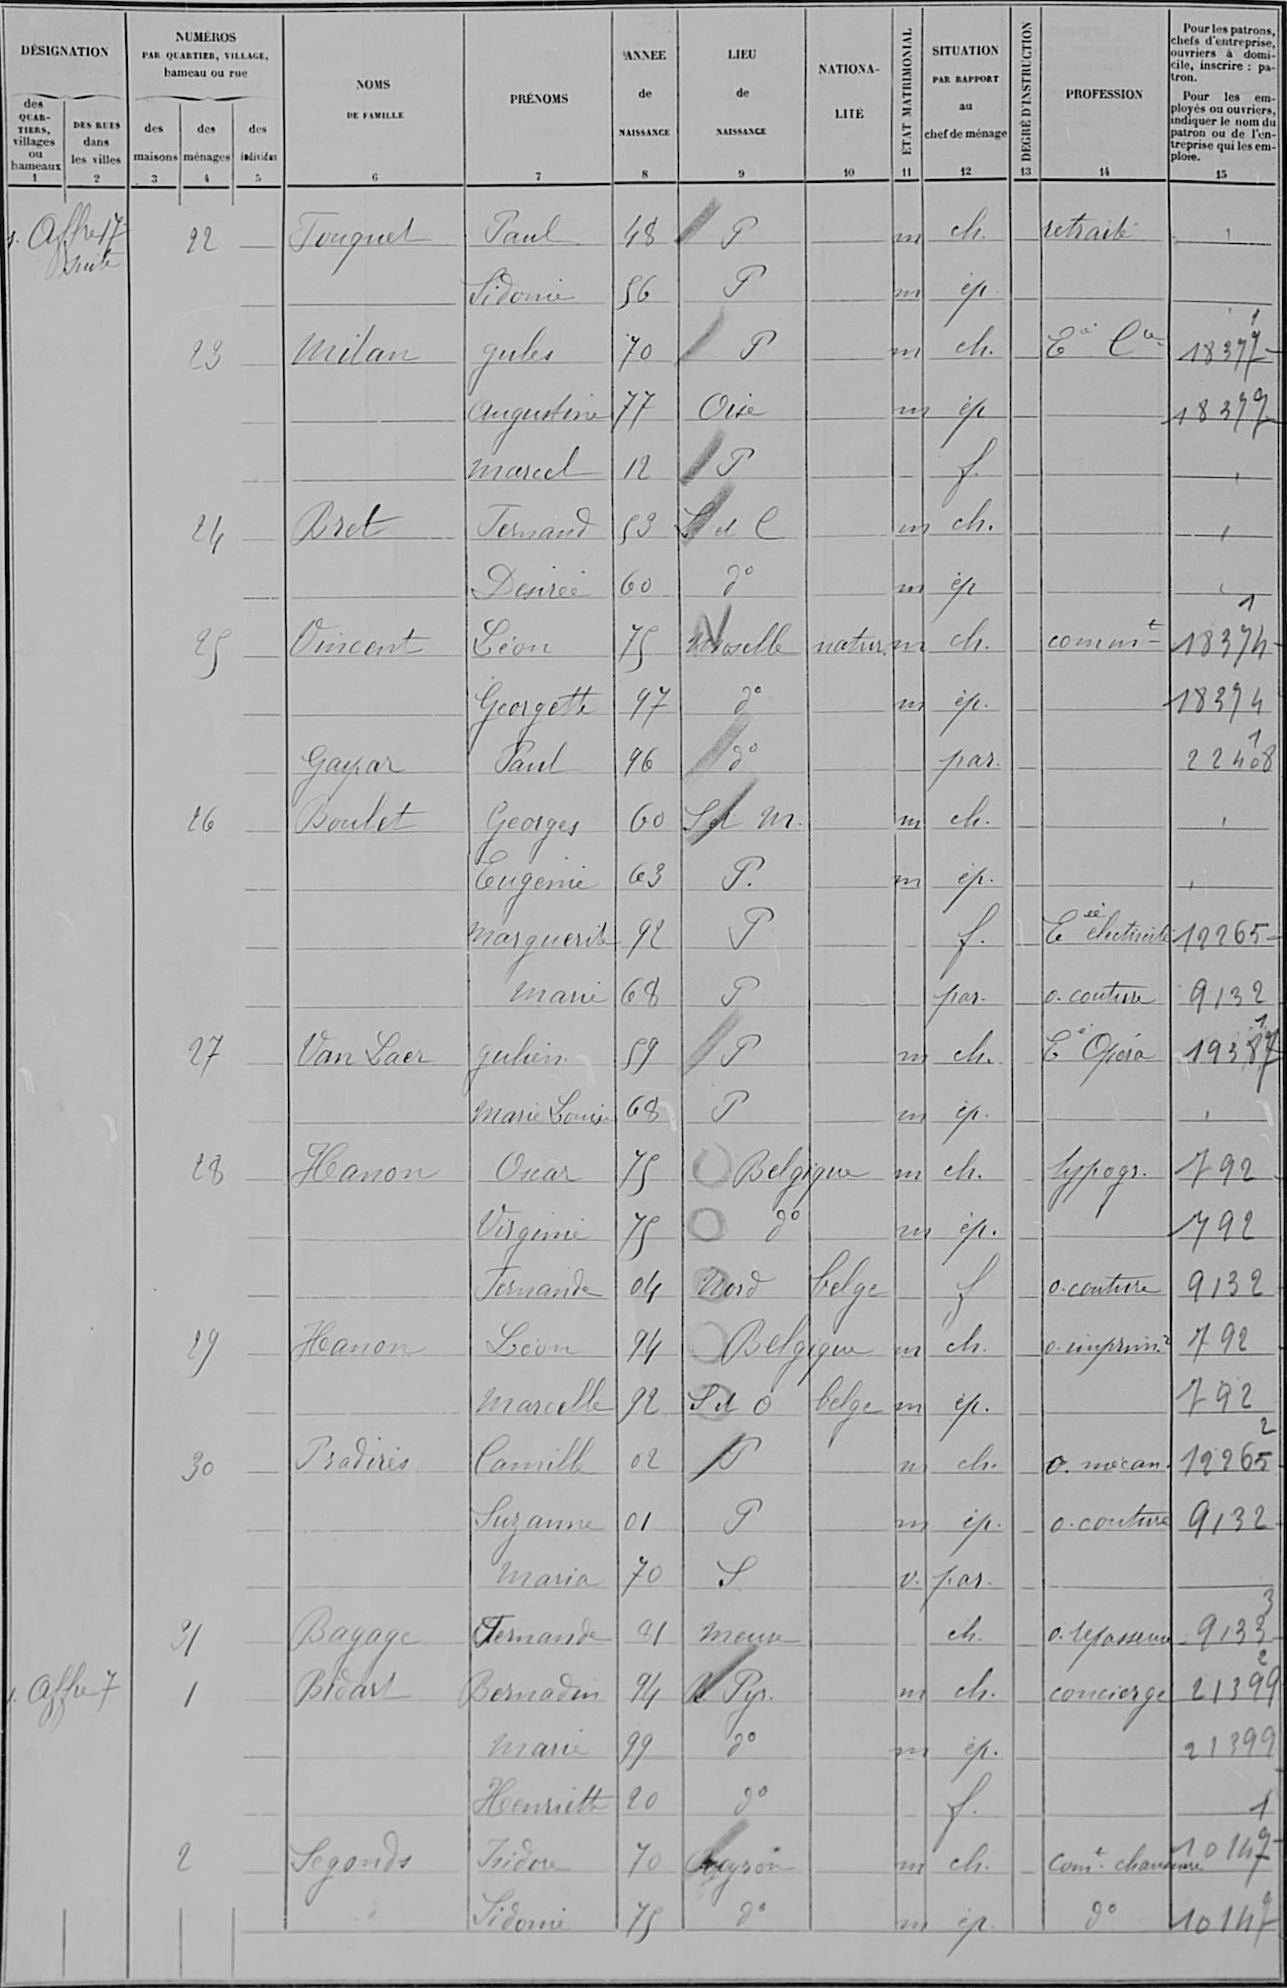Fouquet/Paul/48/P/¤/m/ch /¤/retraité/¤
¤/Sidonie/56/P/¤/m/ép/¤/¤/¤
Milan/Jules/70/P/¤/m/ch /¤/E Cce/18377
¤/Augustine/77/oise/¤/m/ép/¤/¤/18377
¤/Marcel/12/P/¤/¤/f /¤/¤/¤
Brot/Fernand/53/S et L/¤/m/ch /¤/¤/¤
¤/Désirée/60/d°/¤/m/ép /¤/¤/1
Vincent/Léon/75/Moselle/natur/m/ch /¤/commt/18374
¤/Georgette/97/d°/¤/m/ép /¤/¤/18374
Gaipar/Paul/96/d°/¤/¤/par /¤/¤/22408
Boulet/Georges/60/S et m/¤/m/ch /¤/¤/¤
¤/Eugénie/63/P /¤/m/ép /¤/¤/¤
¤/Marguerite/92/P/¤/¤/f /¤/Eée electricité/12265
¤/Marie/68/P/¤/¤/par /¤/o couture/9132
Van Laer/Julien/59/P/¤/m/ch /¤/Eé Opéra/19387
¤/Marie Louise/68/P/¤/m/ép /¤/¤/¤
Hanon/Oscar/75/Belgique/¤/m/ch /¤/hypogr /792
¤/Virginie/75/d°/¤/m/ép /¤/¤/792
¤/Fernande/04/Nord/Belge/¤/f/¤/o couture/9132
Hanon/Léon/94/Belgique/¤/m/ch /¤/o imprim r/792
¤/Marcelle/92/S et O/belge/m/ép /¤/¤/7922
Pradiris/Camille/02/P/¤/m/ch /¤/o mecan /12265
¤/Suzanne/01/P/¤/m/ép /¤/o couture/9132
¤/Maria/70/S/¤/v /par /¤/¤/¤
Bagage/Fernande/81/meuse/¤/¤/ch /¤/o repasseuse/9133
Bidart/Bernadin/94/B Pyr/¤/m/ch /¤/concierge/21399
¤/Marie/99/d°/¤/m/ép /¤/¤/21399
¤/Henriette/20/d°/¤/¤/f /¤/¤/1
Segonds/Isidore/70/Aveyron/¤/m/ch /¤/comt chaussure/10147
¤/Sidonie/75/d°/¤/m/ép /¤/d°/10147
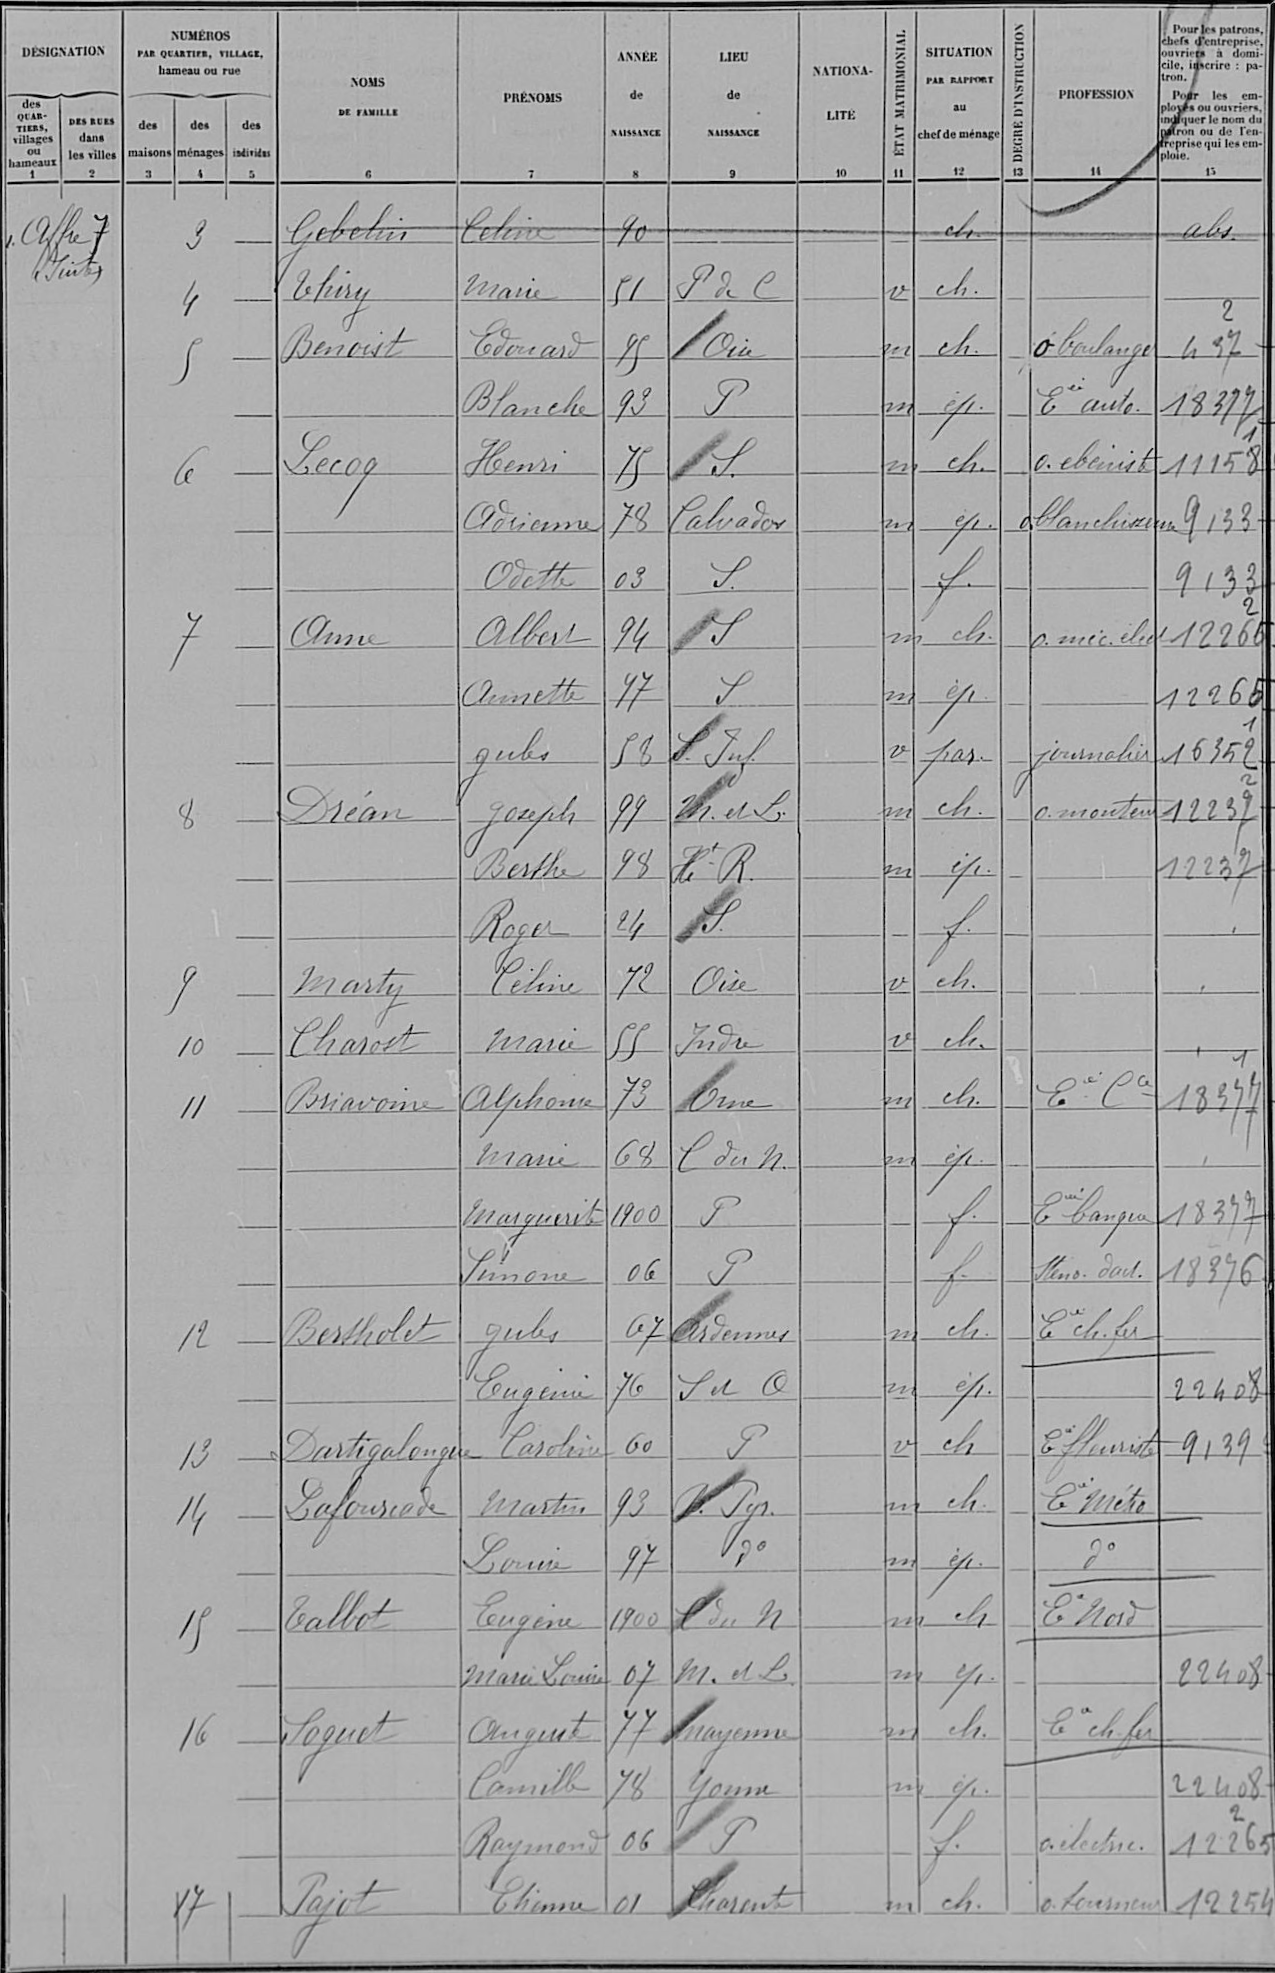Gebelin/Celine/90/¤/¤/¤/ch /¤/¤/abs
Vhiry/Marie/51/P de C/¤/v/ch /¤/¤/2
Benoist/Edouard/95/Oise/¤/m/ch /¤/o boulanger/437
¤/Blanche/93/P/¤/m/ep /¤/E auto/18377
Lecoq/Henri/75/S/¤/m/ch /¤/o ebeniste/11158
¤/Adrienne/78/Calvados/¤/m/ep /¤/o blanchisseuse/9133
¤/Odette/03/S /¤/¤/f /¤/¤/9133
Anne/Albert/94/S/¤/m/ch /¤/o méc elect/12266
¤/Annette/97/S/¤/m/ép /¤/¤/12265
¤/Jules/58/S Inf /¤/v/par /¤/journalier/16352
Dréan/Joseph/99/M et L /¤/m/ch /¤/o monteur/12237
¤/Berthe/98/Ht-R/¤/m/ép /¤/¤/12237
¤/Roger/24/S /¤/¤/f /¤/¤/¤
Marty/Céline/72/Oise/¤/v/ch /¤/¤/¤
Charost/Marie/55/Indre/¤/v/ch /¤/¤/¤
Briavoine/Alphonse/73/Orne/¤/m/ch /¤/Eé Cce/18377
¤/Marie/68/C du N/¤/m/ép /¤/¤/¤
¤/Marguerite/1900/P/¤/¤/f /¤/E banque/18377
¤/Simone/06/P/¤/¤/f /¤/Steno dact /18376
Bertholet/Jules/07/Ardennes/¤/m/ch /¤/E ch fer/¤
¤/Eugénie/76/S et O/¤/m/ép /¤/¤/22408
Dartigalongue/Caroline/60/P/¤/v/ch/¤/E fleuriste/9139
Lafourcade/Martin/93/B Pyr/¤/m/ch /¤/E Métro/¤
¤/Louise/97/d°/¤/m/Ep /¤/d°/¤
Talbot/Eugène/1900/C du N/¤/m/ch/¤/E Nord/¤
¤/Marie Louise/07/M et L /¤/m/ep /¤/¤/22408
Soguet/Auguste/77/mayenne/¤/m/ch /¤/E ch fer/¤
¤/Camille/78/Yonne/¤/m/ép /¤/¤/22408
¤/Raymond/06/P/¤/¤/f /¤/o electric /12265
Pajot/Etienne/01/Charente/¤/m/ch /¤/o tourneur/12254